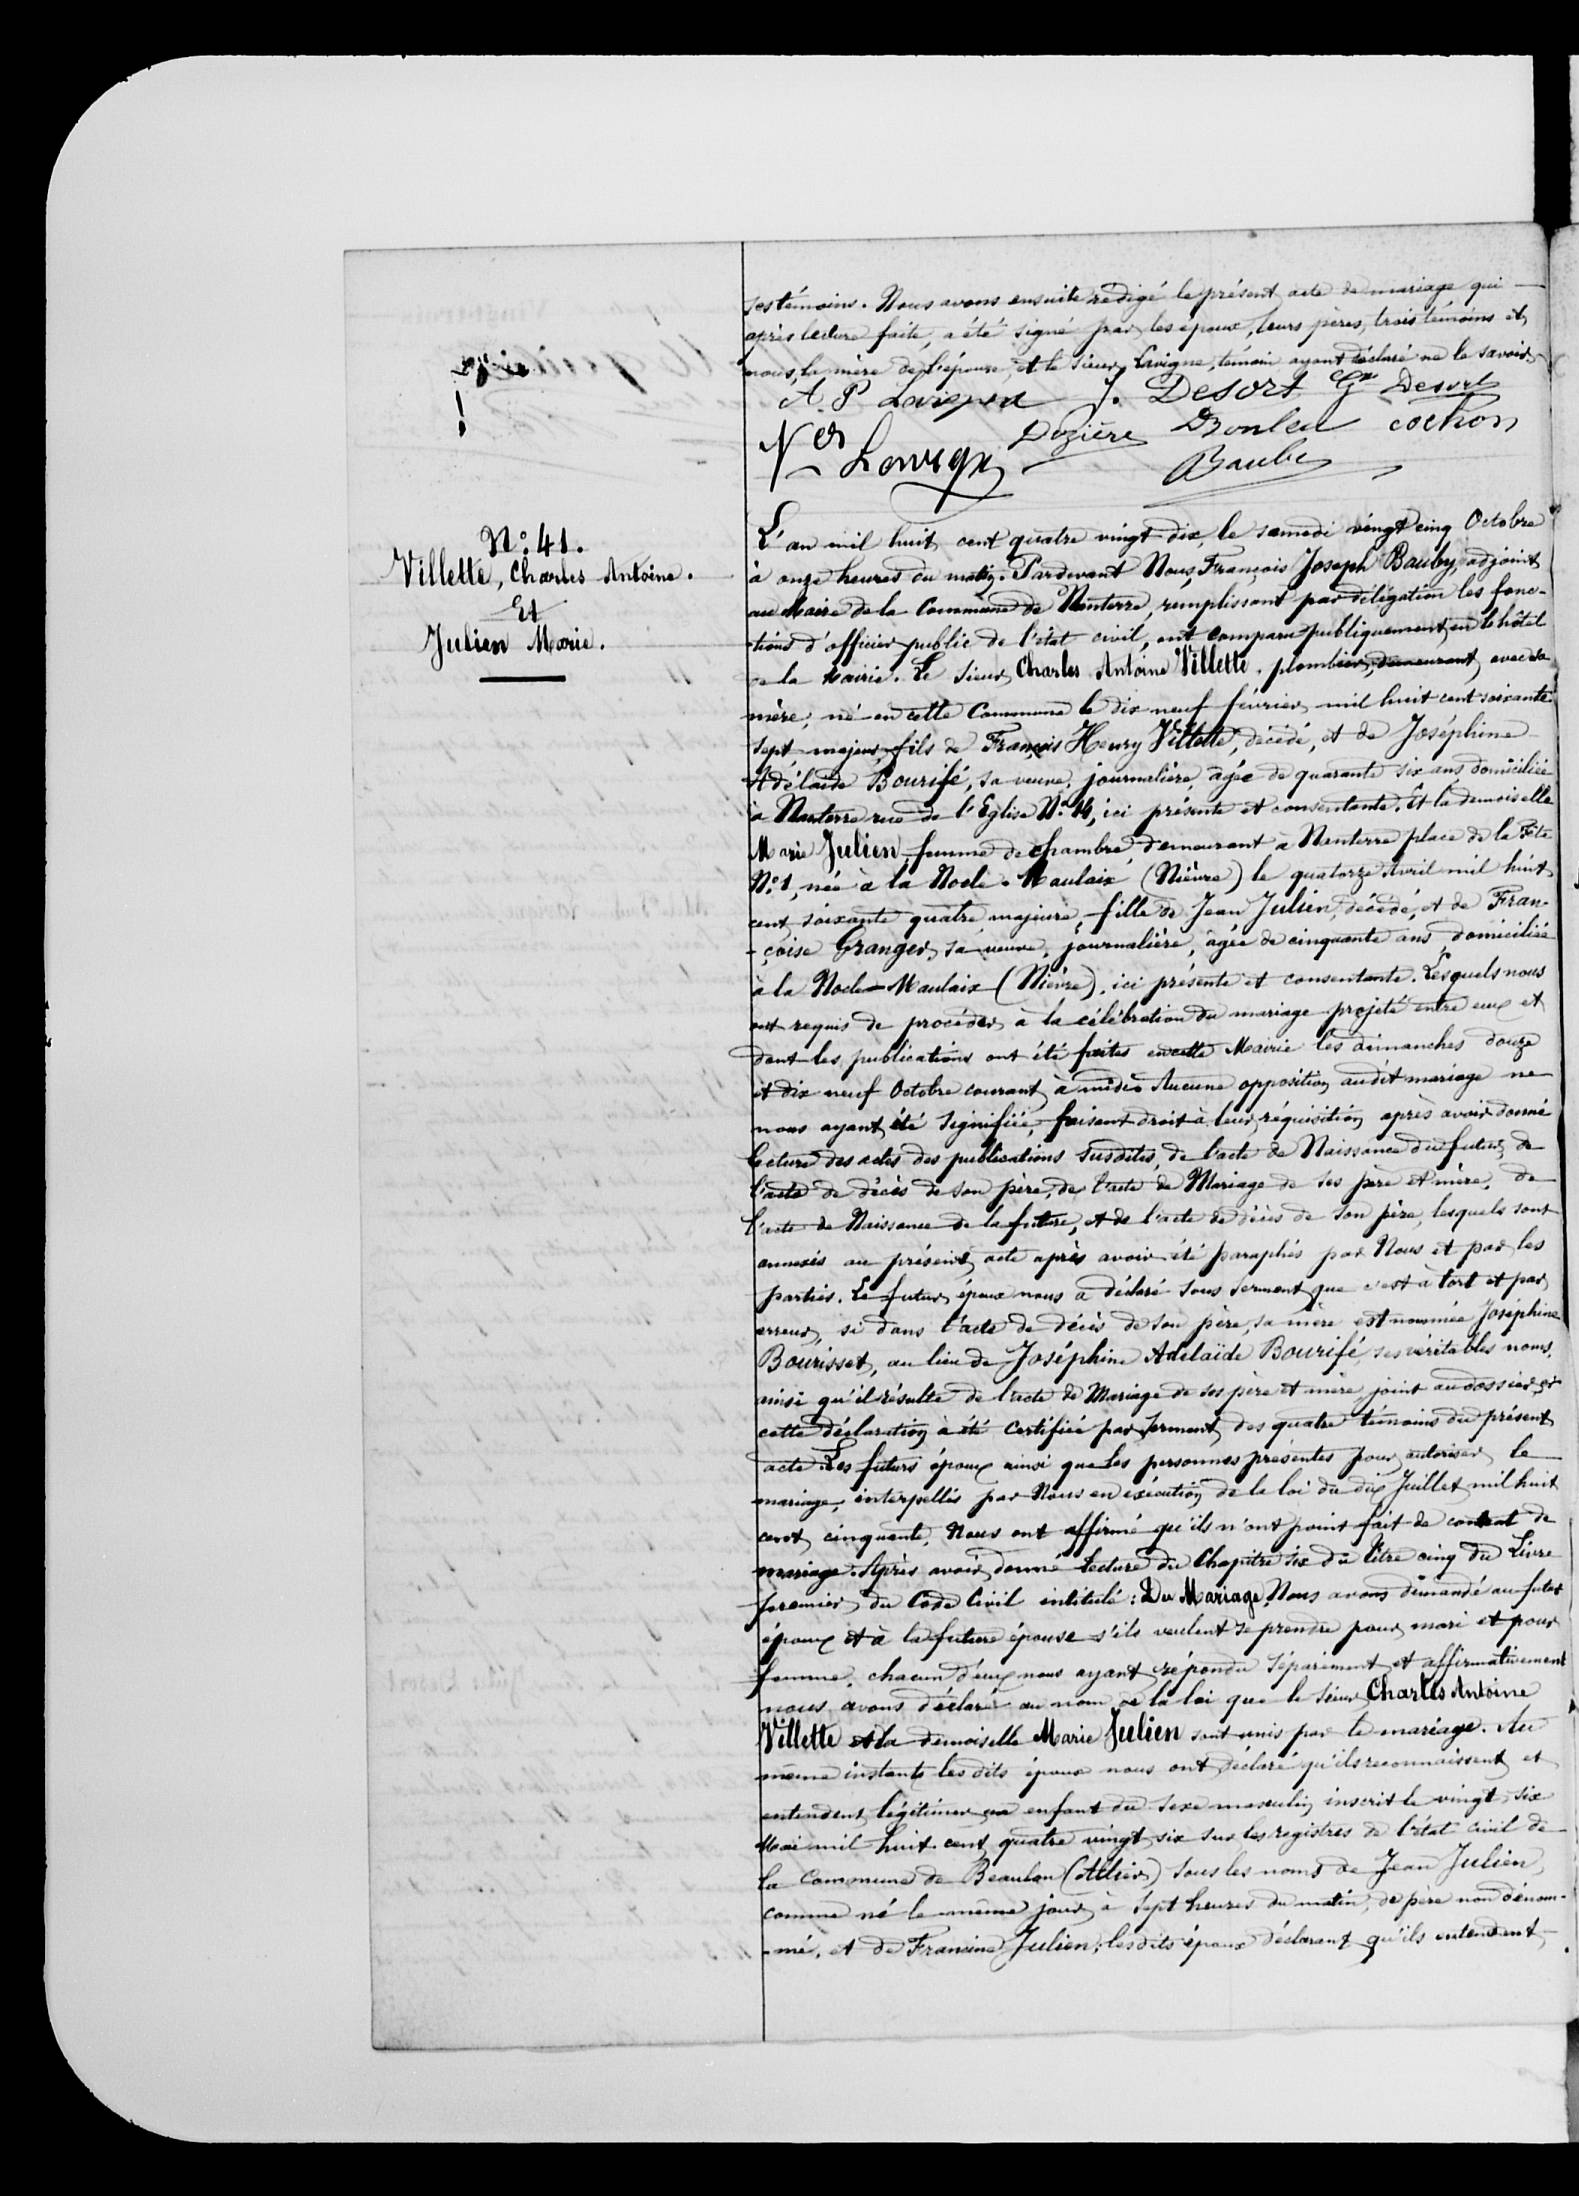ses témoins. Nous avons ensuite rédigé le présent acte de mariage qui -
après lecture faite a été signé par les époux, leurs pères, trois témoins et
nous, la mère de l'épouse et la soeur Lavigne, témoin ayant déclaré ne le savoir.
N: 41
Villette, Charles Antoine.
Et
Julien Marie.
L'an mil huit cent quatre vingt dix, le samedi vingt cinq Octobre
à onze heures du matin Par devant Nous, François Joseph Bauby, adjoint
au Maire de la Commune de Nanterre remplissant par délégation les fonc-
-tions d'officier public de l'état civil, ont comparu publiquement en l'hôtel
de la Mairie. Le Sieur Charles Antoine Villette, plombier, demeurant, avec sa
mère, né en cette commune le dix neuf février mil huit cent soixante
sept majeurs, fils de François Henry Vilette, décédé, et de Joséphine -
Héloise Bourifé, sa veuve, journalière agée de quarante six ans domiciliée
à Nanterre rue de l'Eglise N°: 14, ici présents et consentante, et la demoiselle
Marie Julienne femme de chambre, demeurant à Nanterre place de la Fête
N°:1, née à la Nocle-Maulaix (Nièvre) le quatorze Avril mil huit
cent soixante quatre majeure, fille de Jean Julien décédé, et de Fran
-çoise Granges sa veuve, jounalière, âgée de cinquante ans domiciliée
à la Nocle-Maulaix (Nièvre), ici présente et consentante. Lesquels nous
ont requis de procédés à la célébration du mariage projeté entre eux et
dont les publications ont été faites en cette Mairie les dimanches douze
et dix neuf octobre courant à midi Aucune opposition audit mariage ne
nous ayant été signifiée, faisant droit à leur réquisition après avoir donné
lecture des actes des publications susdites, de l'acte de Naissance dufuture, de
l'acte de décès du père, de l'acte de Mariage de ses père et mère, de
l'acte de Naissance de la future, et de l'acte de décès de son père, lesquels sont
annexés au présent acte après avoir été paraphés par Nous et par les
parties. Les futurs époux nous a déclaré sous serment que c'est à tort et par
erreur, si dans l'acte de décès de son père, sa mère est nommée Joséphine
Baurisset, au lieu de Joséphine Adelaïde Bourifé, ses véritables noms,
ainsi qu'il résulte de l'acte de Mariage de ses père et mère joint au dossiers et
cette déclaration a été certifiée par serment des quatres témoins du présent
acte. Les futurs époux ainsi que les personnes présentes pour autoriser le
mariage, interpellés par Nous en exécution de la loi du dix Juillet mil huit
cent cinquante Nous ont affirmé qu'ils n'ont point fait de contrat de
mariage. Après avoir donné lexture du Chapitre six du livre cinq du Livre
premier du Code Civil intitulé: Du Mariage. Nous avons demandé au futur
époux et à la future épouse s'ils veulent se prendre pour mari et pour
femme, chacun d'eux nous ayant répondu séparément et affirmativement
nous avons déclaré au nom de la loi que le Sieur Charles Antoine
Villette et la demoiselle Marie Julien sont unis par le mariage. Au
même instants les dits époux nous ont déclaré qu'ils reconnaissent et
entendent légitime un enfant du sexe masculin inscrit le vingt six
mai mil huit cent quatre vingt six sur les registres de l'état civil de
la Commune de Beaulon (Allier) sous les noms de Jean Julien
comme né le même jour à sept heures du matin, de père non dénom-
-mé, et de Francine Julien; les dits époux déclarent qu'ils entendent
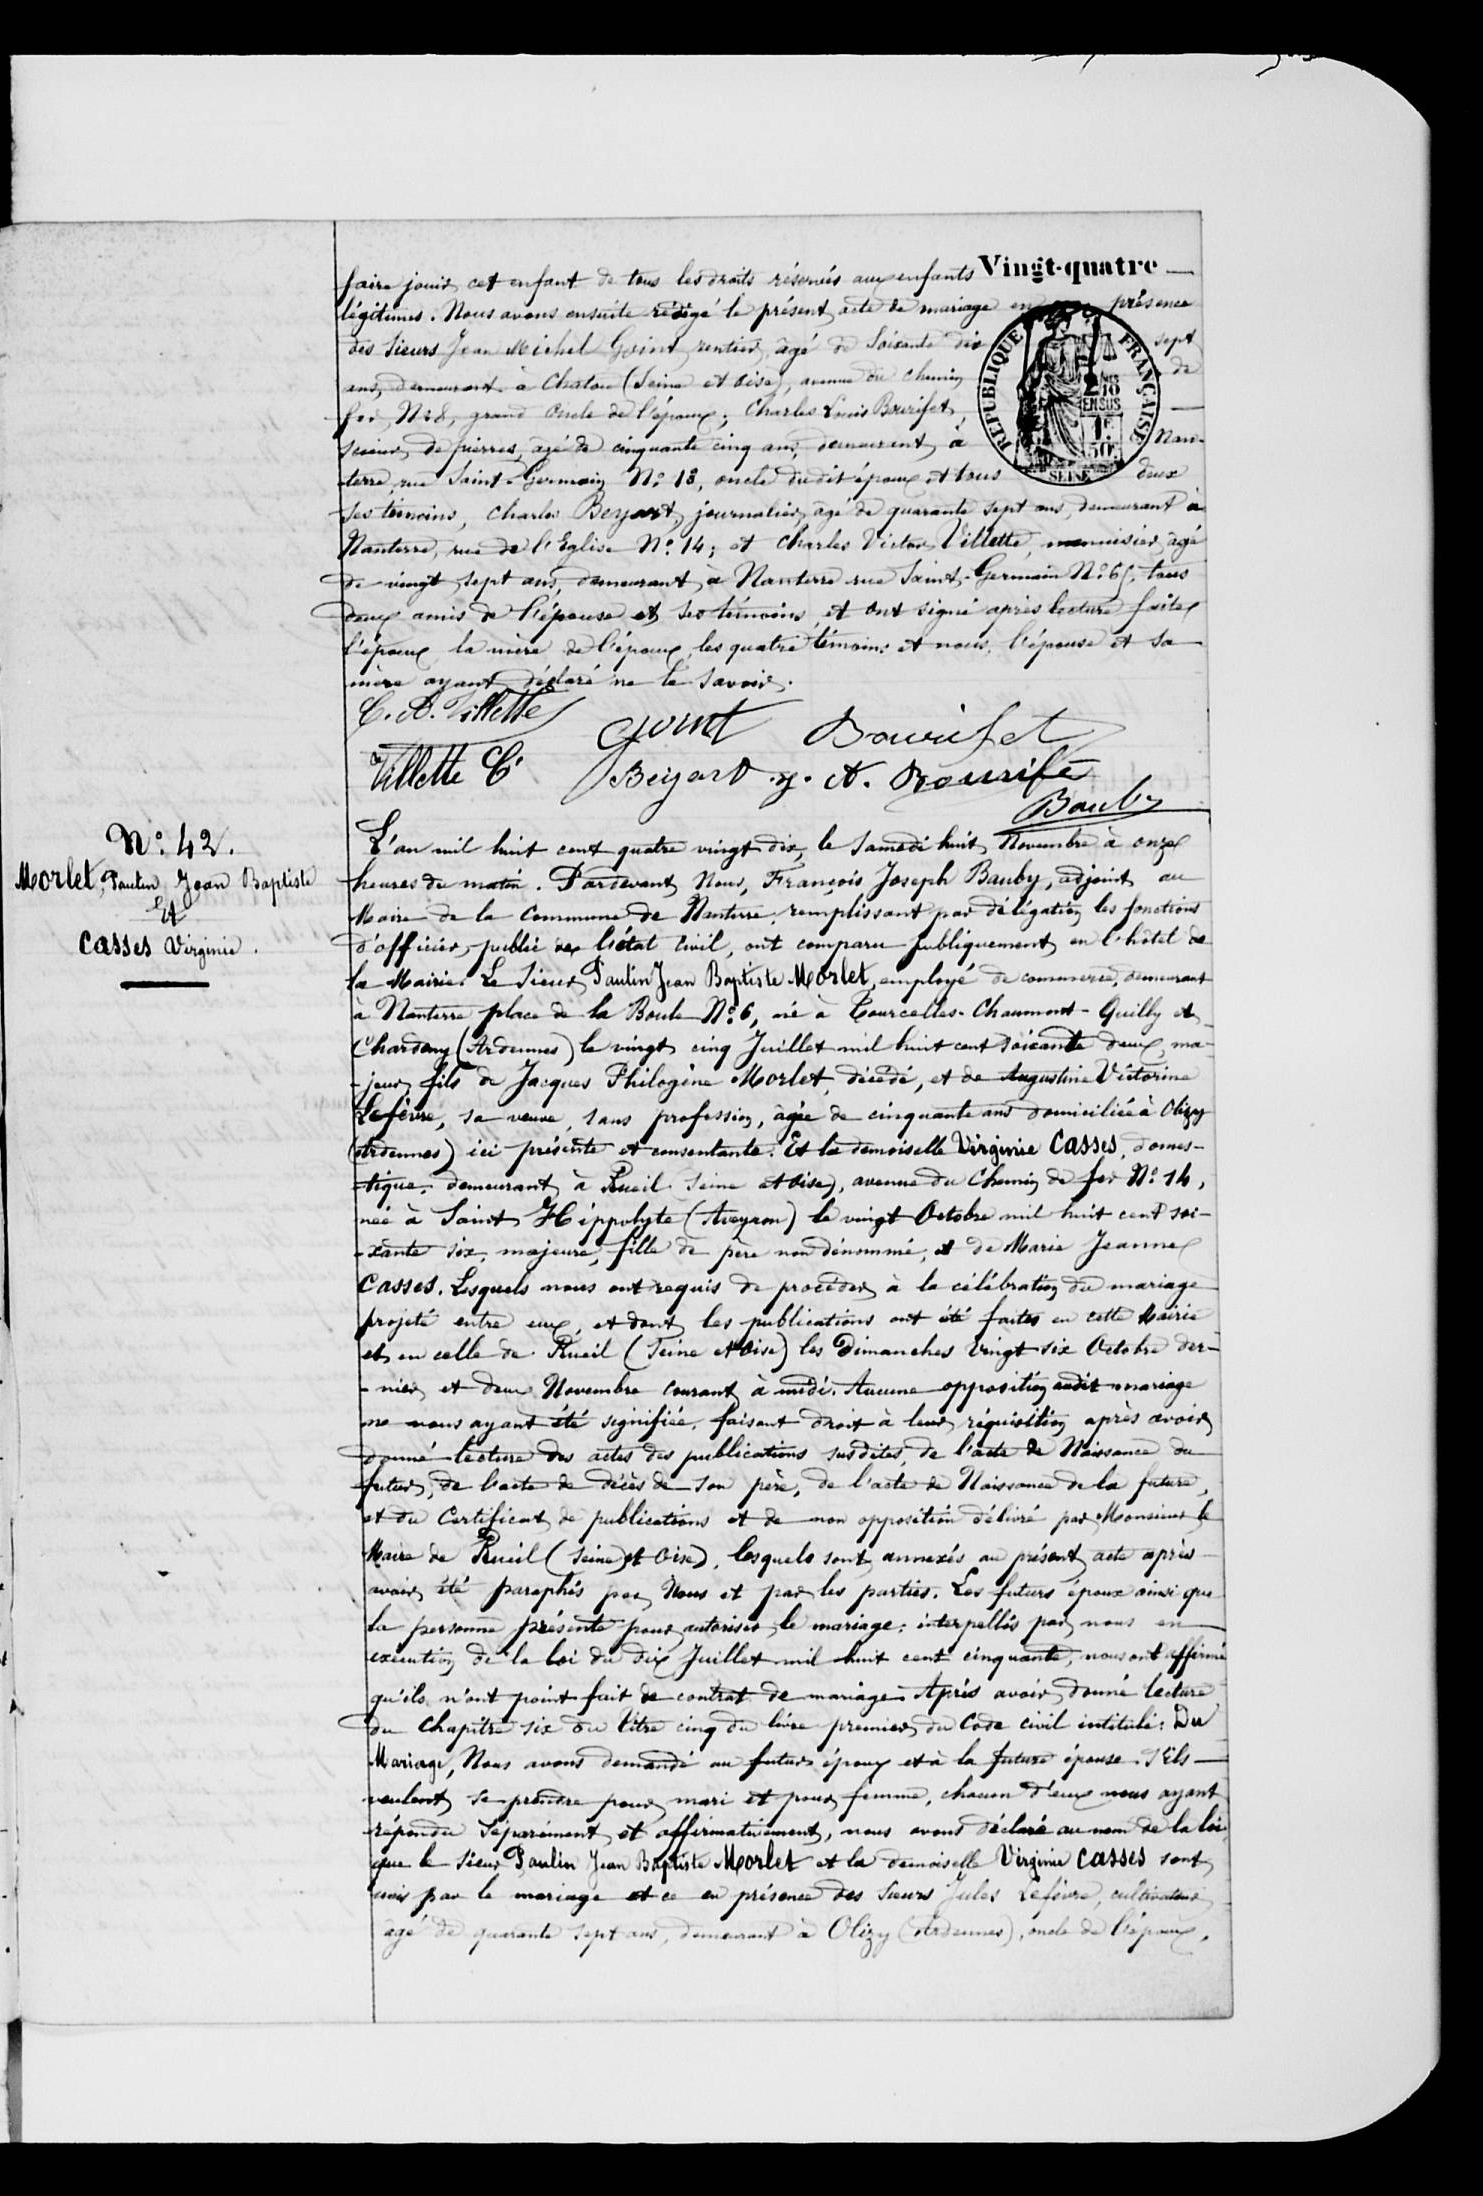faire jouir cet enfant de tous les droits réservés aux enfants Vingt-quatre
légitimes. Nous avons ensuite rédigé le présent acte de mariage en présence
des Sieurs Jean Michel Goin rentiers âgé de soixant dix sept
ans demeurant à Châton (Seine et Oise) avenue du chemin de
fer, N:8, grand Oncle de l'époux; Charles Louis Bourifet
scieur de pierres, âgé de cinquante cinq ans demeurant à Nan
terre rue Saint-Germain N°18, oncle du dit époux et tous deux
ses témoins, Charles Beyant, journalier âgé de quarante sept ans, demeurant à
Nanterre, rue de l'Eglise N°14, et Charles Victore Villette, menuisier, âgé
de vingt sept ans, demeurant à Nanterre rue Saint-Germain N: 69 tous
deux amis de l'épouse et ses témoins et ont signé après lecture faite de
l'époux, la mère de l'épouse, les quatre témoin et nous, l'épouse et sa
mère ayant déclaré ne le savoir.
N°43
Morlet Pantin Jean Baptiste
et
Casses Virginie
L'an mil huit cent quatre vingt dix, le samedi huit Novembre à onze
heures du matin. Par devant nous, François Joseph Bauby, adjoint au
Maire de la Commune de Nanterre remplissant par délégation les fonctions
d'officier, officier de l'état civil, ont comparu publiquement en l'hôtel de
la Mairie le Sieur Paulin Jean Baptise Morlet, employé de commerce, demeurant
à Nanterre place de la Boule N: 6, né à Coucelles-Chaumont-Guilly et
Chadeny (Ardennes) le vingt cinq Juillet mil huit cent soixante-deux ma-
-jeurs fils de Jacques Philogène Morlet décédé, et de Augustine Victorine
Lefévre, sa veuve, 1 ans profession, âgée de cinquante ans domiciliée à Oligny
(Ardennes) ici présente et consentante. Et la demoiselle Virginie casses, domes-
-tique, demeurant à Reuil (Seine et Oise), avenue du Chemin de fer N°14,
née à Saint Hippolyte (Aveyron) le vingt Octobre mil huit cent soi-
xante six majeure, fille de père non dénomé, et de Marie Jeanne
Casses. Lesquels nous ont requis de procédé à la célébration du mariage
projeté entre eux, et dont les publications ont été faite en cette Mairie
et en celle de Reuil (Seine et Oise) le dimanches vingt-dix Octobre der-
-nier et deux Novembre courant à midi. Aucune opposition audit mariage
ne nous ayant été signifié, faisant droit à leur réquisition après avoir
donné lecture des actes des publications sus dites, de l'acte de Naissance des
futurs, de l'acte de décès de son père, de l'acte de Naissance de la futur
et du Certificat de publications et de mon opposition délivré par Monsieur le
Maire de Reuil (Seine et Oise) lesquels sont annexés au présent acte après
avoir été paraphés par Nous et par les parties. Les futurs époux ainsi que
la personne présente pour autorisé le mariage: interpellés par nous en
execution de la loi du dix Juillet mil huit cent cinquante, nous ont affirmé
qu'ils n'ont point fait de contrat de mariage Après avoir donné lecture
du chapitre six du livre cinq du livre premier du Code Civil intitulé: du
Mariage, Nous avons demandé au futur époux et à la futur épouse s'ils-
veulent se prendre pour mari et pour femme, chacun d'eux nous ayant
répondu séparément et affirmativement, nous avons déclaré au nom de la loi
que le Sieur, Paulin Jean Baptiste Morlet et la demoiselle Virgine Casses sont
unis par le mariage et en présence des soeurs Jules Lefèvre, cultivateurs,
âgé de quarante sept ans, demeurant à Olizy (Dromes), oncle de l'époux,